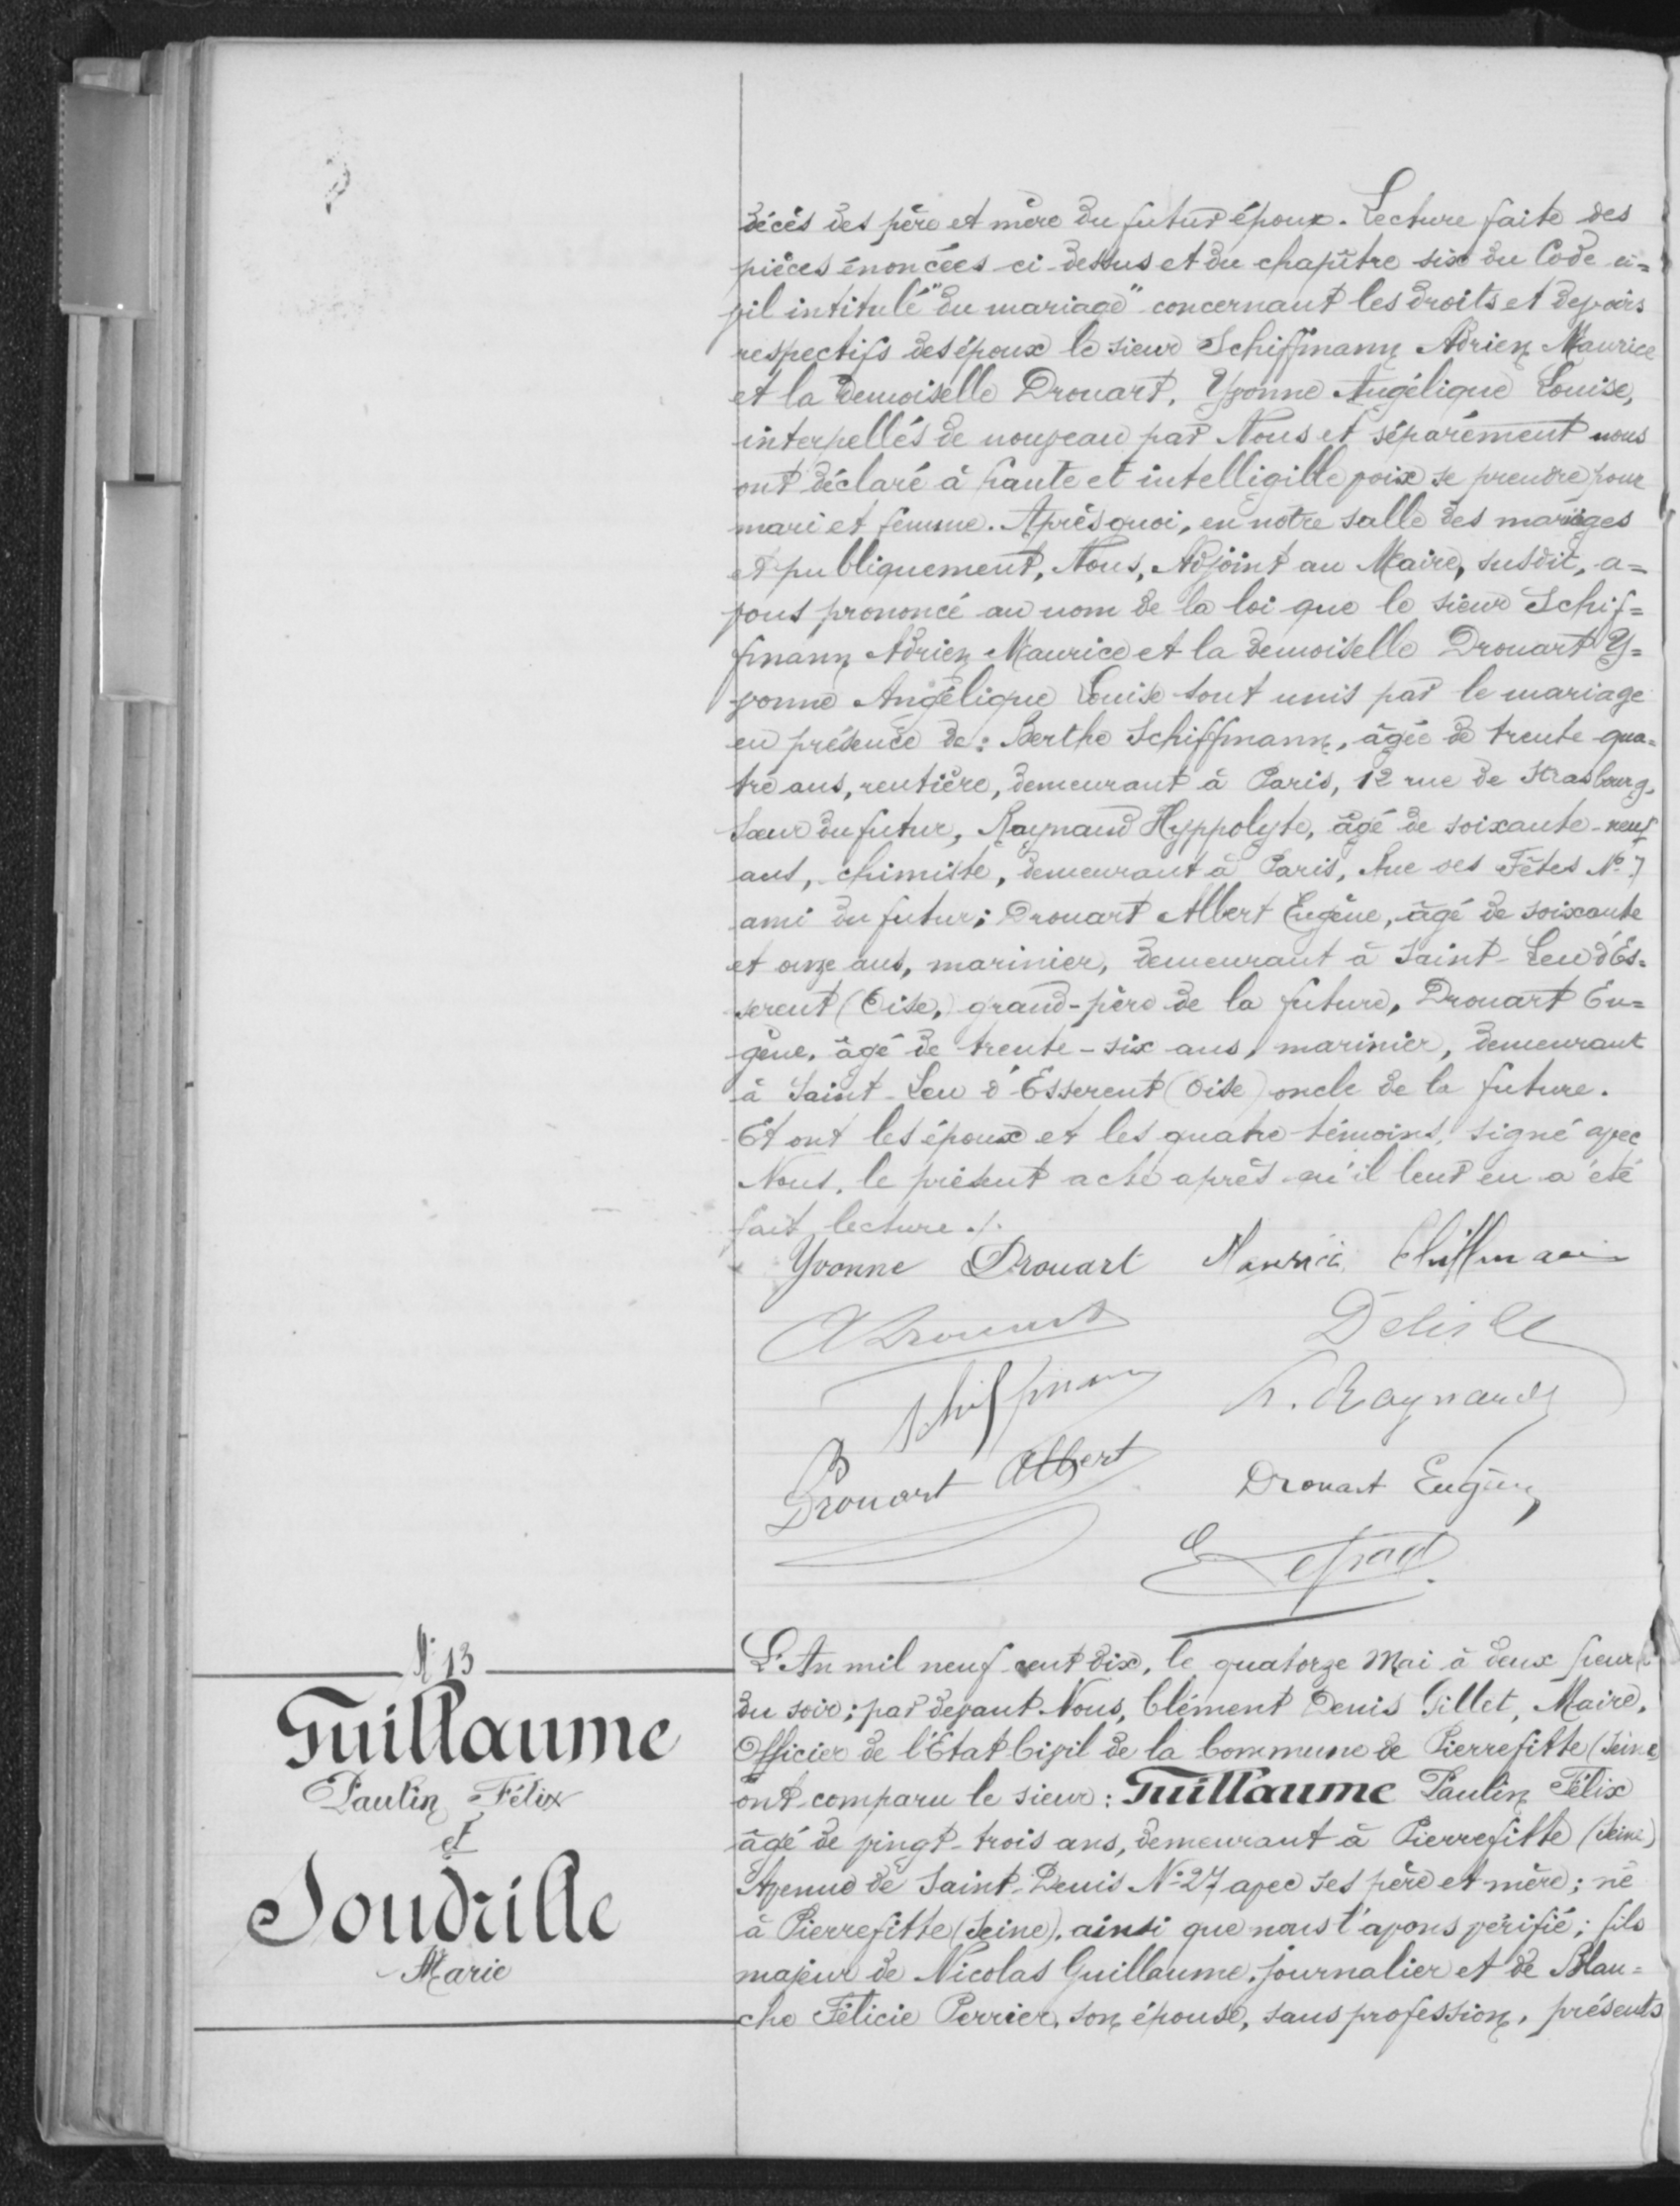décès des père et mère du futur époux. Lecture faite des
pièces énoncées ci-dessus et du chapitre six du Code ci
vil intitulé "du mariage" concernant les droits et devoirs
respectifs des époux le sieur schiffmann.; Adrien Mauice
et la Demoiselle Dronart, Yvonne, Angélique Louise,
interpellés de nouveau par Nous et séparément nous
ont déclaré à haute et intelligible voix se prendre pour
mari et femme. Après quoi, en notre salle des mariages
et publiquement, Nous, Adjoint au Maire, susdit, a -
nous prononcé au nom de la loi que le sieur Schif-
fman Briez Maurice et la demoiselle Drouart Y-
vone Angélique Louise sont unis par le mariage
en présence de: Berthe Schiffmann, âgée de trente qua-
tre ans, rentière, demeurant à Paris, 12 rue de Strasbourg,
soeur du futur, Raynaud Hyppolyte, âgé de soixante -neuf
ans, chimiste, demeurant à Paris, Rue des Fêtes n°7
ami du futur; Drouart Albert Eugène, âgé de soixante
et onze ans, marinier, demeurant à Saint Leu d'Es
serent (Oise), grand-père de la future, Drouart Eu-
gène, âgé de trente-six ans, marinier, demeurant
à Saint-Leu d'Esserent (Oise) oncle de la future.
Et ont les époux et les quatre témoins signé avec
Nous, le présent acte après qu'il leur en a été
fait lecture ./.
N°13
Guillaume
Paulin Félix
et
Soudrille
Marie
L'An mil neuf cent dix, le quatorze Mai à deux heures
su soir; par devant Nous, Clément Denis Gillet, Maire,
Officier de l'Etat Civil de la Commune de Pierrefitte (Seine)
ont comparu le sieur: Guillaume Paulin Félix
âgé de vingt-trois-ans, demeurant à Pierrefitte (Seine)
Avenue de Saint-Denis N°27 avec ses père et mère; né
à Pierrefitte (Seine), ainsi que nous l'ayons vérifié; fils
majeur de Nicolas Guillaume, journalier et de Blan=
che Félicie Perrier, son épouse, sans profession, présents
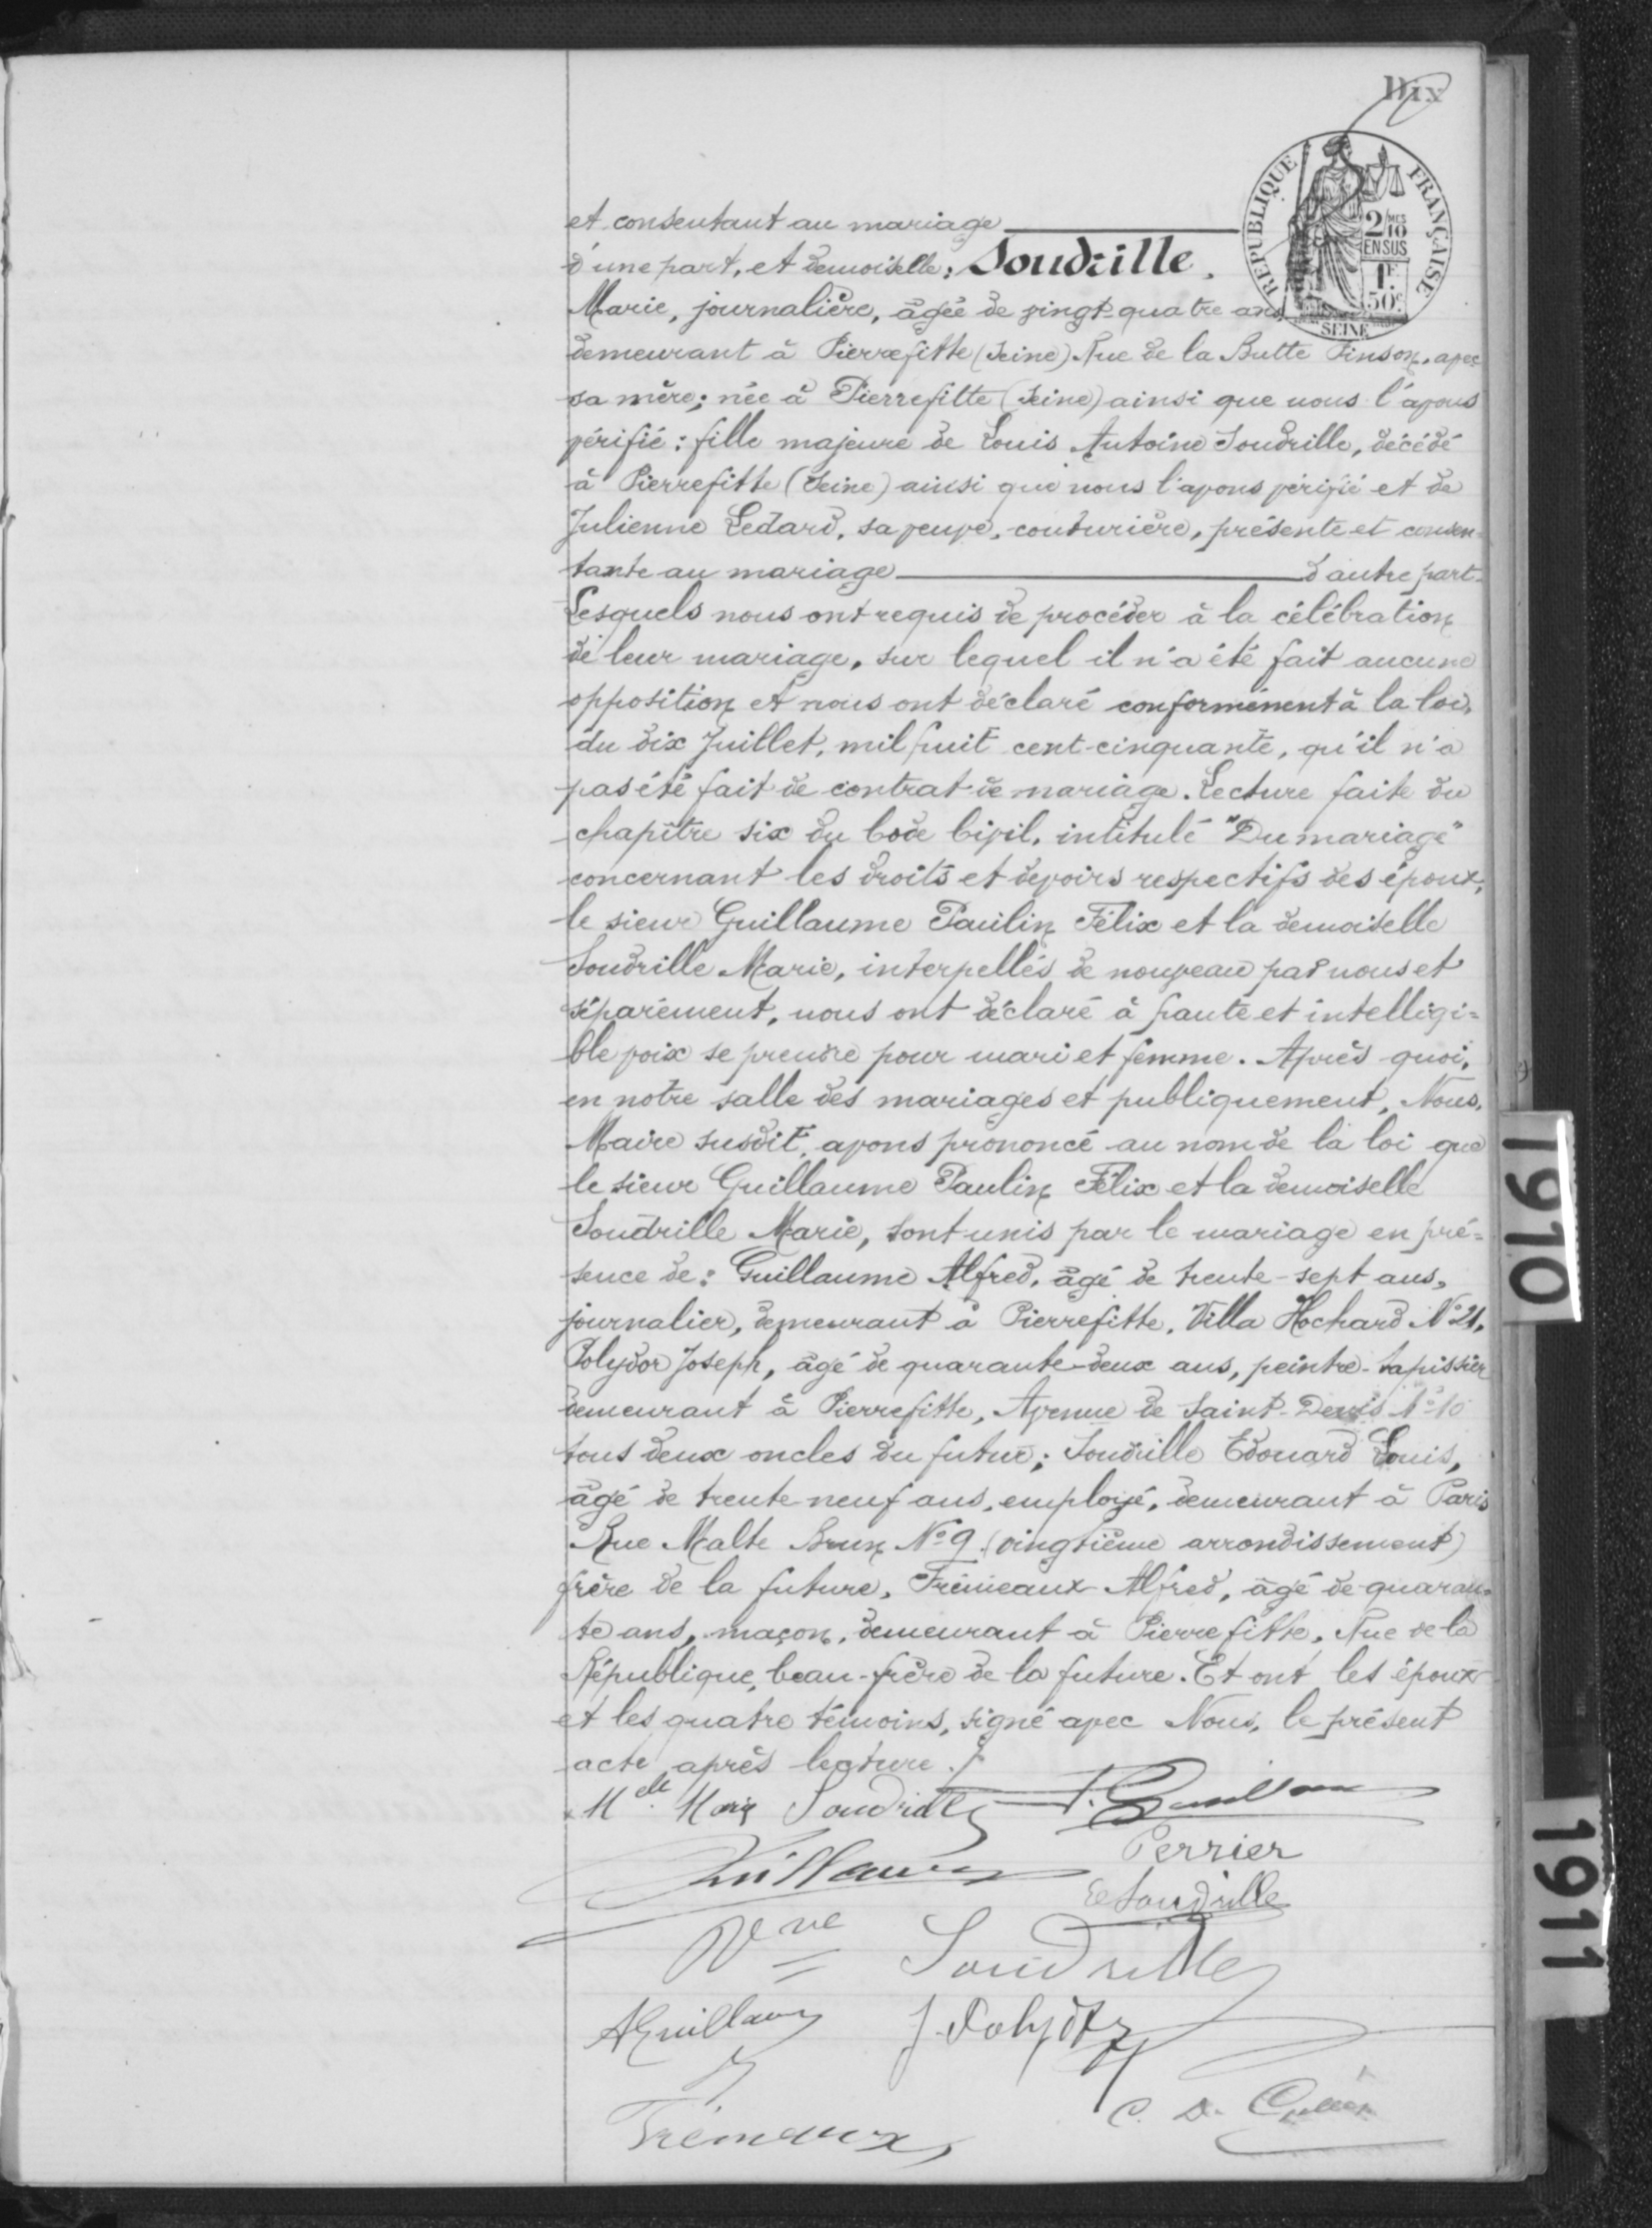et consentant au mariage
d'une part, et demoiselle: Soudrille,
Marie, journalière, âgée de vingt-quatre ans
demeurant à Pierrefitte (Seine) Rue de la Butte Pinson, avec
sa mère; née à Pierrefitte (Seine) ainsi que nous l'ayons
vérifié: fille majeure de Louis Antoine Soudrille, décédé
à Pierrefitte (Seine) ainsi que nous l'avons vérifié et de
Julienne Ledard, sa veuve, couturière, présente et consen-
tante au mariage d'autre part
Lesquels nous ont requis de procéder à la célébration
de leur mariage, sur lequel il n'a été fait aucune
opposition et nous ont déclaré conformément à la loi,
du dix juillet, mil huit cent cinquante, qu'il n'a
pas été fait de contrat de mariage. Lecture faite du
chapitre six du Code Civil, intitulé "Du mariage"
concernant les droits et devoirs respectifs des époux,
le sieur Guillaume Paulin Félix et la demoiselle
Soudrille Marie, interpellés de nouveau par nous et
séparément, nous ont déclaré à haute et intelligi=
ble voix se prendre pour mai et femme. Après quoi
en notre salle des mariages et publiquement, Nous,
Maire susdit, avons prononcé au nom de la loi que
le sieur Guillaume Paulin Félix et la demoiselle
Soudrille Marie, sont unis par le mariage en pré=
sence de: Guillaume Alfred, âgé de trente-sept ans,
journalier, demeurant à Pierrefitte, Villa hochard N°21,
Polydor Joseph, âgé de quarante-deux ans, peintre tapissier
demeurant à Pierrefitte, Avenue de Saint-Denis n°10
tous deux oncles du futur; Soudrille Edouard Louis,
âgé de trente neuf ans, employé, demeurant à Paris
Rue Malte Boune N°9 (vingtième arrondissement)
frère de la future, Frénieaux Alfred, âgé de quaran
te ans, maçon, demeurant à Pierrefitte, Rue de la
République, beau-frère de la future. Et ont les époux
et les quatre témoins, signé avec Nous, le présent
acte, après lecture ./.